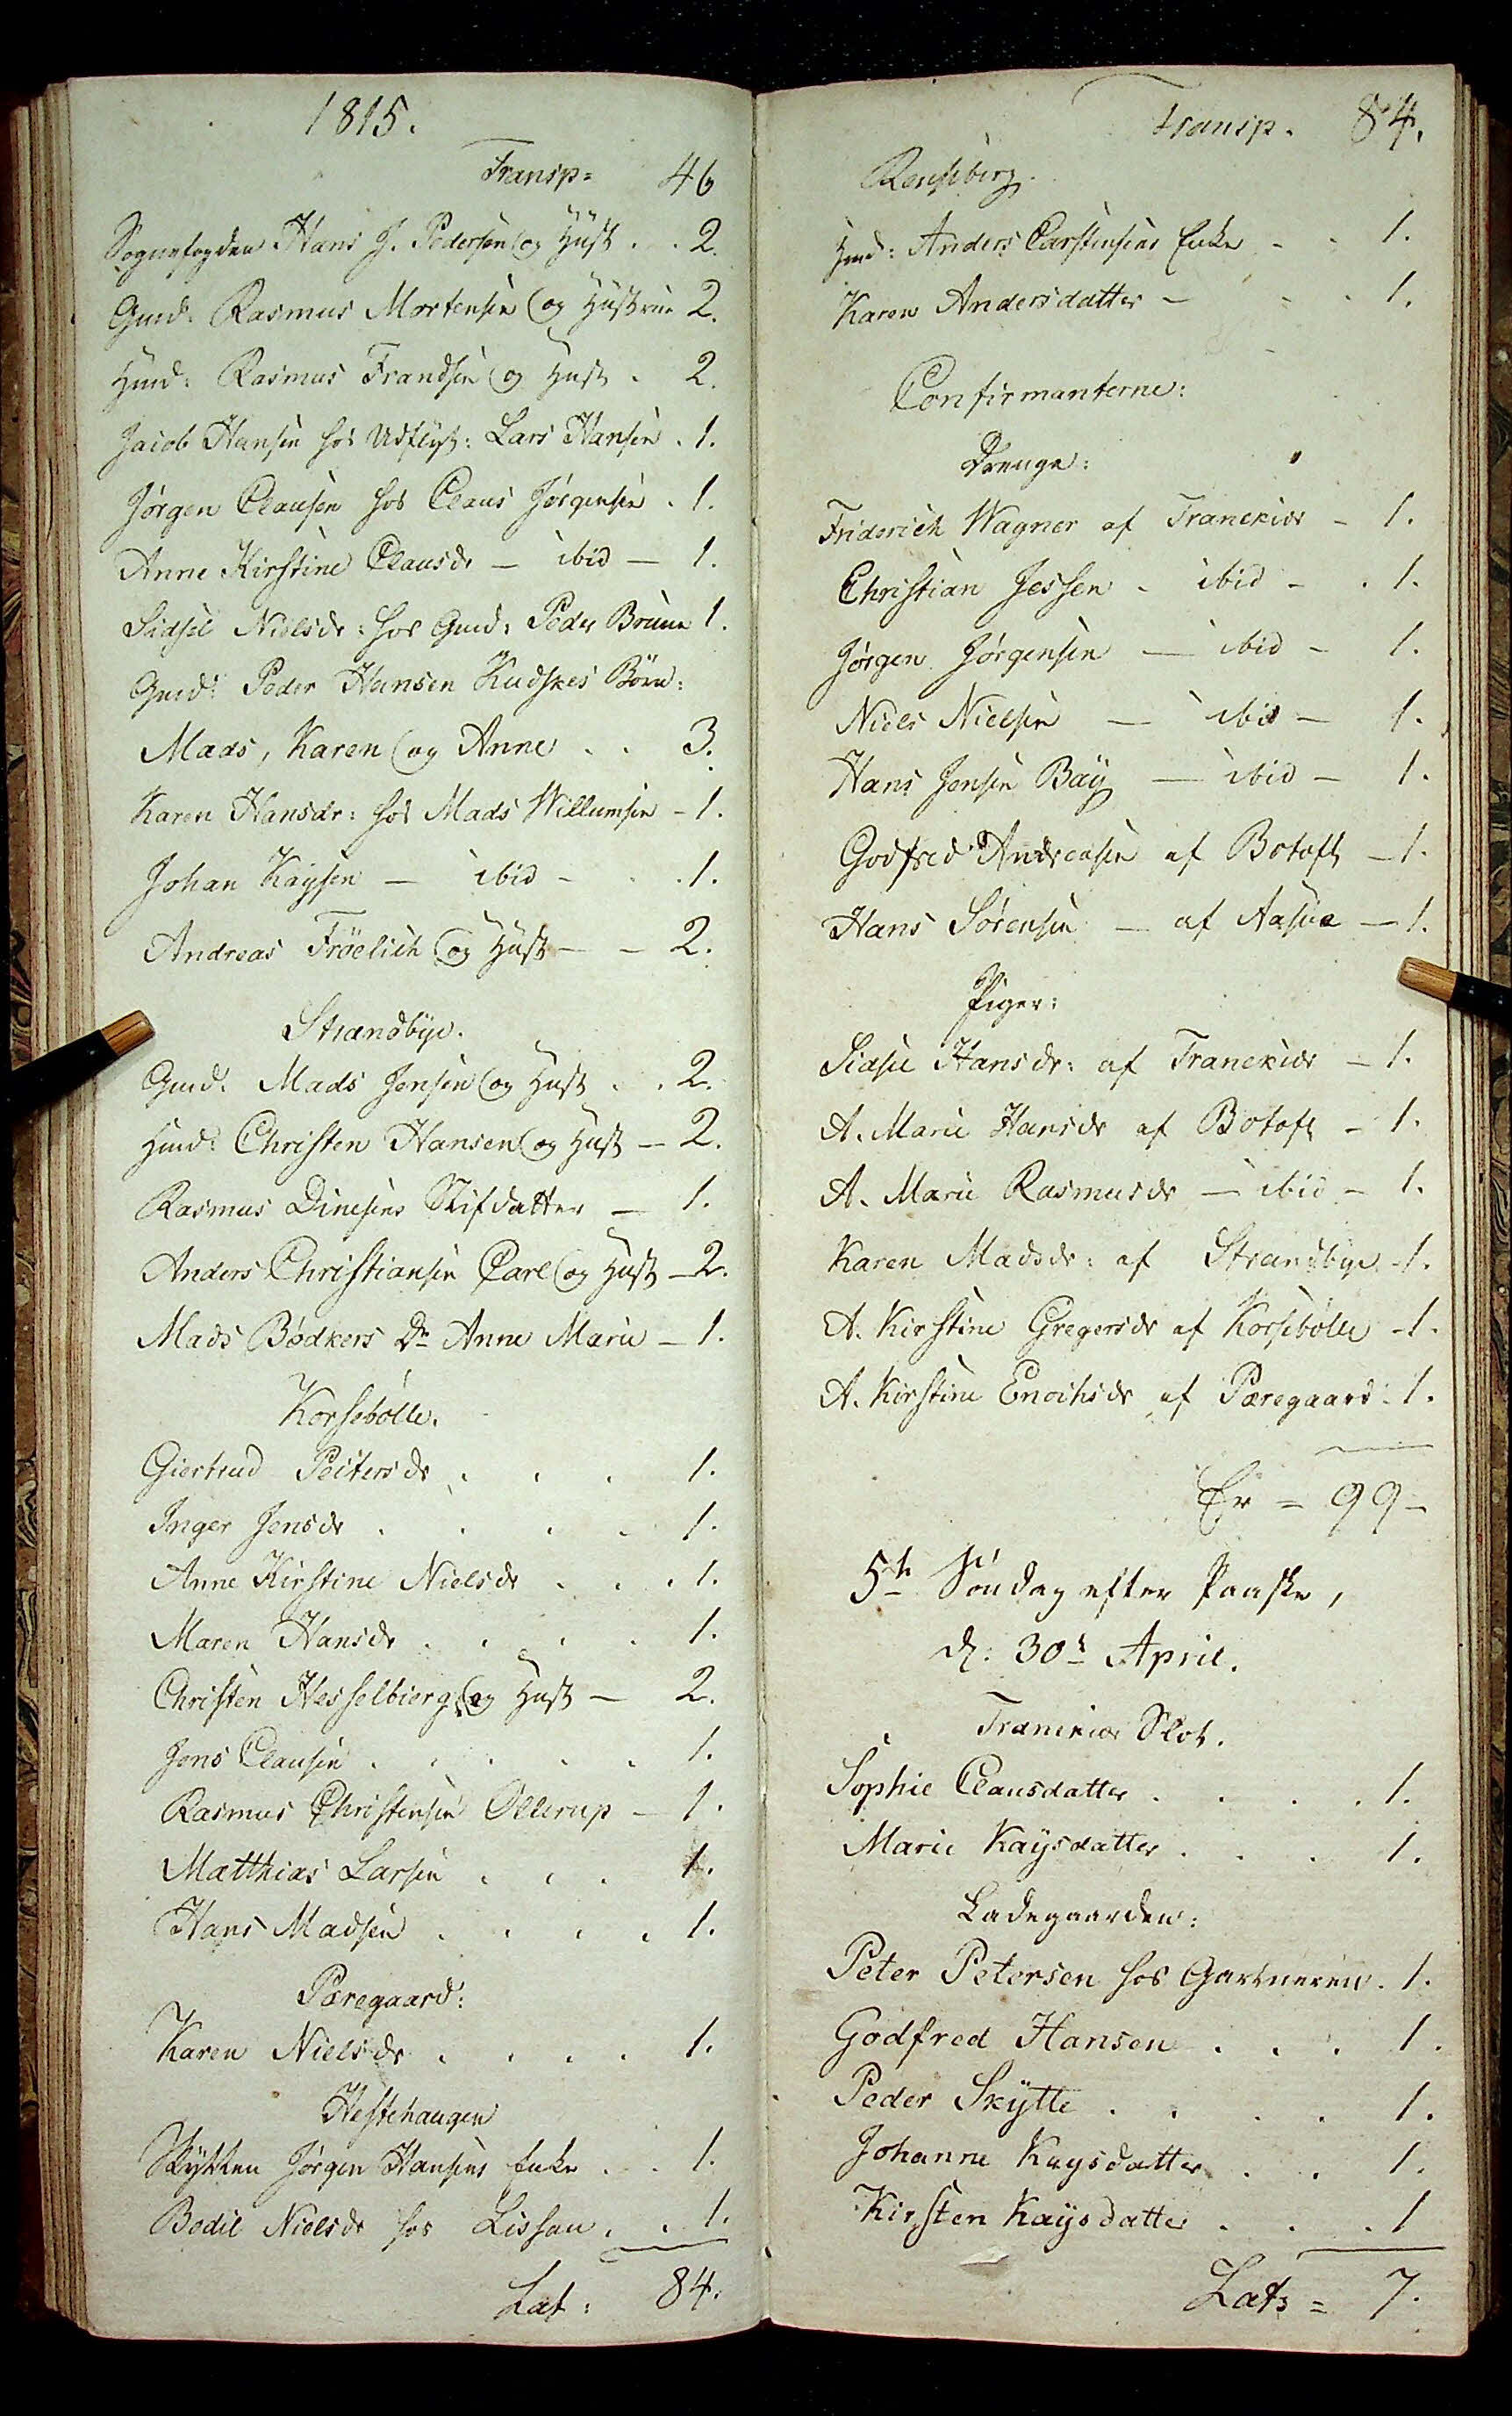1815.
Transp= 46
Sognefogden Hans J. Pedersen og Hust . . 2.
Gmd: Rasmus Mortensen og Hustrue 2.
Hmd: Rasmus Frandsen og Hust . 2.
Jacob Hansen hos Udflut: Lars Hansen . 1.
Jørgen Clausen hos Claus Jørgensen . 1.
Anne Kirstine Clausdr - ibid - 1.
Sidsel Nielsdr: hos Gmd: Peder Bruun 1.
Gmd: Peder Hansen Kudskes Børn:
Mads, Karen og Anne . . 3.
Karen Hansdr hos Mads Willumsen - 1.
Johan Kaysen - ibid - . .  1.
Andreas Frøclich og Hust - - 2.
Strandbye.
Gmd. Mads Jensen og Hust . . 2.
Hmd: Christen Hansen og Hust - - 2.
Rasmus Dinesens Stifdatter - 1.
Anders Christiansen Carl og Hust - 2.
Mads Bødkers Dr Anne Marie - 1.
Korsebølle.
Giertrud Peitersdr . . . 1.
Inger Jensdr . . . . 1.
Anne Kirstine Nielsdr . . . 1.
Maren Hansdr . . . . 1.
Christen Hesselbierg og Hust - 2.
Jens Clausen . . . . . 1.
Rasmus Christensen Ollerup - 1.
Matthias Larsen . . . 1.
Hans Madsen . . . 1.
Pæregaard:
Karen Nielsdr . . . . 1.
Hestehaugen
Skytten Jørgen Hansens Enke . . 1.
Bodil Nielsdr hos Lissau . . 1.
Lat: 84.
Transp . 84.
Renseberg
Hmd: Anders Carstensens Enke . . 1.
Karen Andersdatter - . . 1.
Confirmanterne:
Drenge:
Friderich Wagner af Tranekiær - 1.
Christian Jensen - ibid -  . 1.
Jørgen Jørgensen - ibid - 1.
Niels Nielsen - ibid - 1.
Hans Jensen Bay - ibid - 1.
Godfred Andreasen af Botoft - 1.
Hans Sørensen - af Aasøe - 1.
Piger:
Sidse Hansdr: af Tranekiær - 1.
A.  Marie Hansdr af Botoft - 1.
A. Marie Rasmusdr - ibid - 1.
Karen Madsdr: af Strandbyr - 1.
A. Kirstine Gregersdr af Korsebølle - 1.
A. Kirstine Enochsdr af Pæregaard - 1.
Er = 99-
5te Søndag efter Paaske,
d: 30te April.
Tranekiær Slot.
Sophie Clausdatter . . . . 1.
Marie Kaysdatter . . . 1.
Ladegaarden.
Peter Petersen hos Gartneren . 1.
Godfred Hansen . . . 1.
Peder Skytte . . . . 1.
Johanne Kaysdatter . . 1.
Kirsten Kaysdatter . . . 1
Lats= 7.
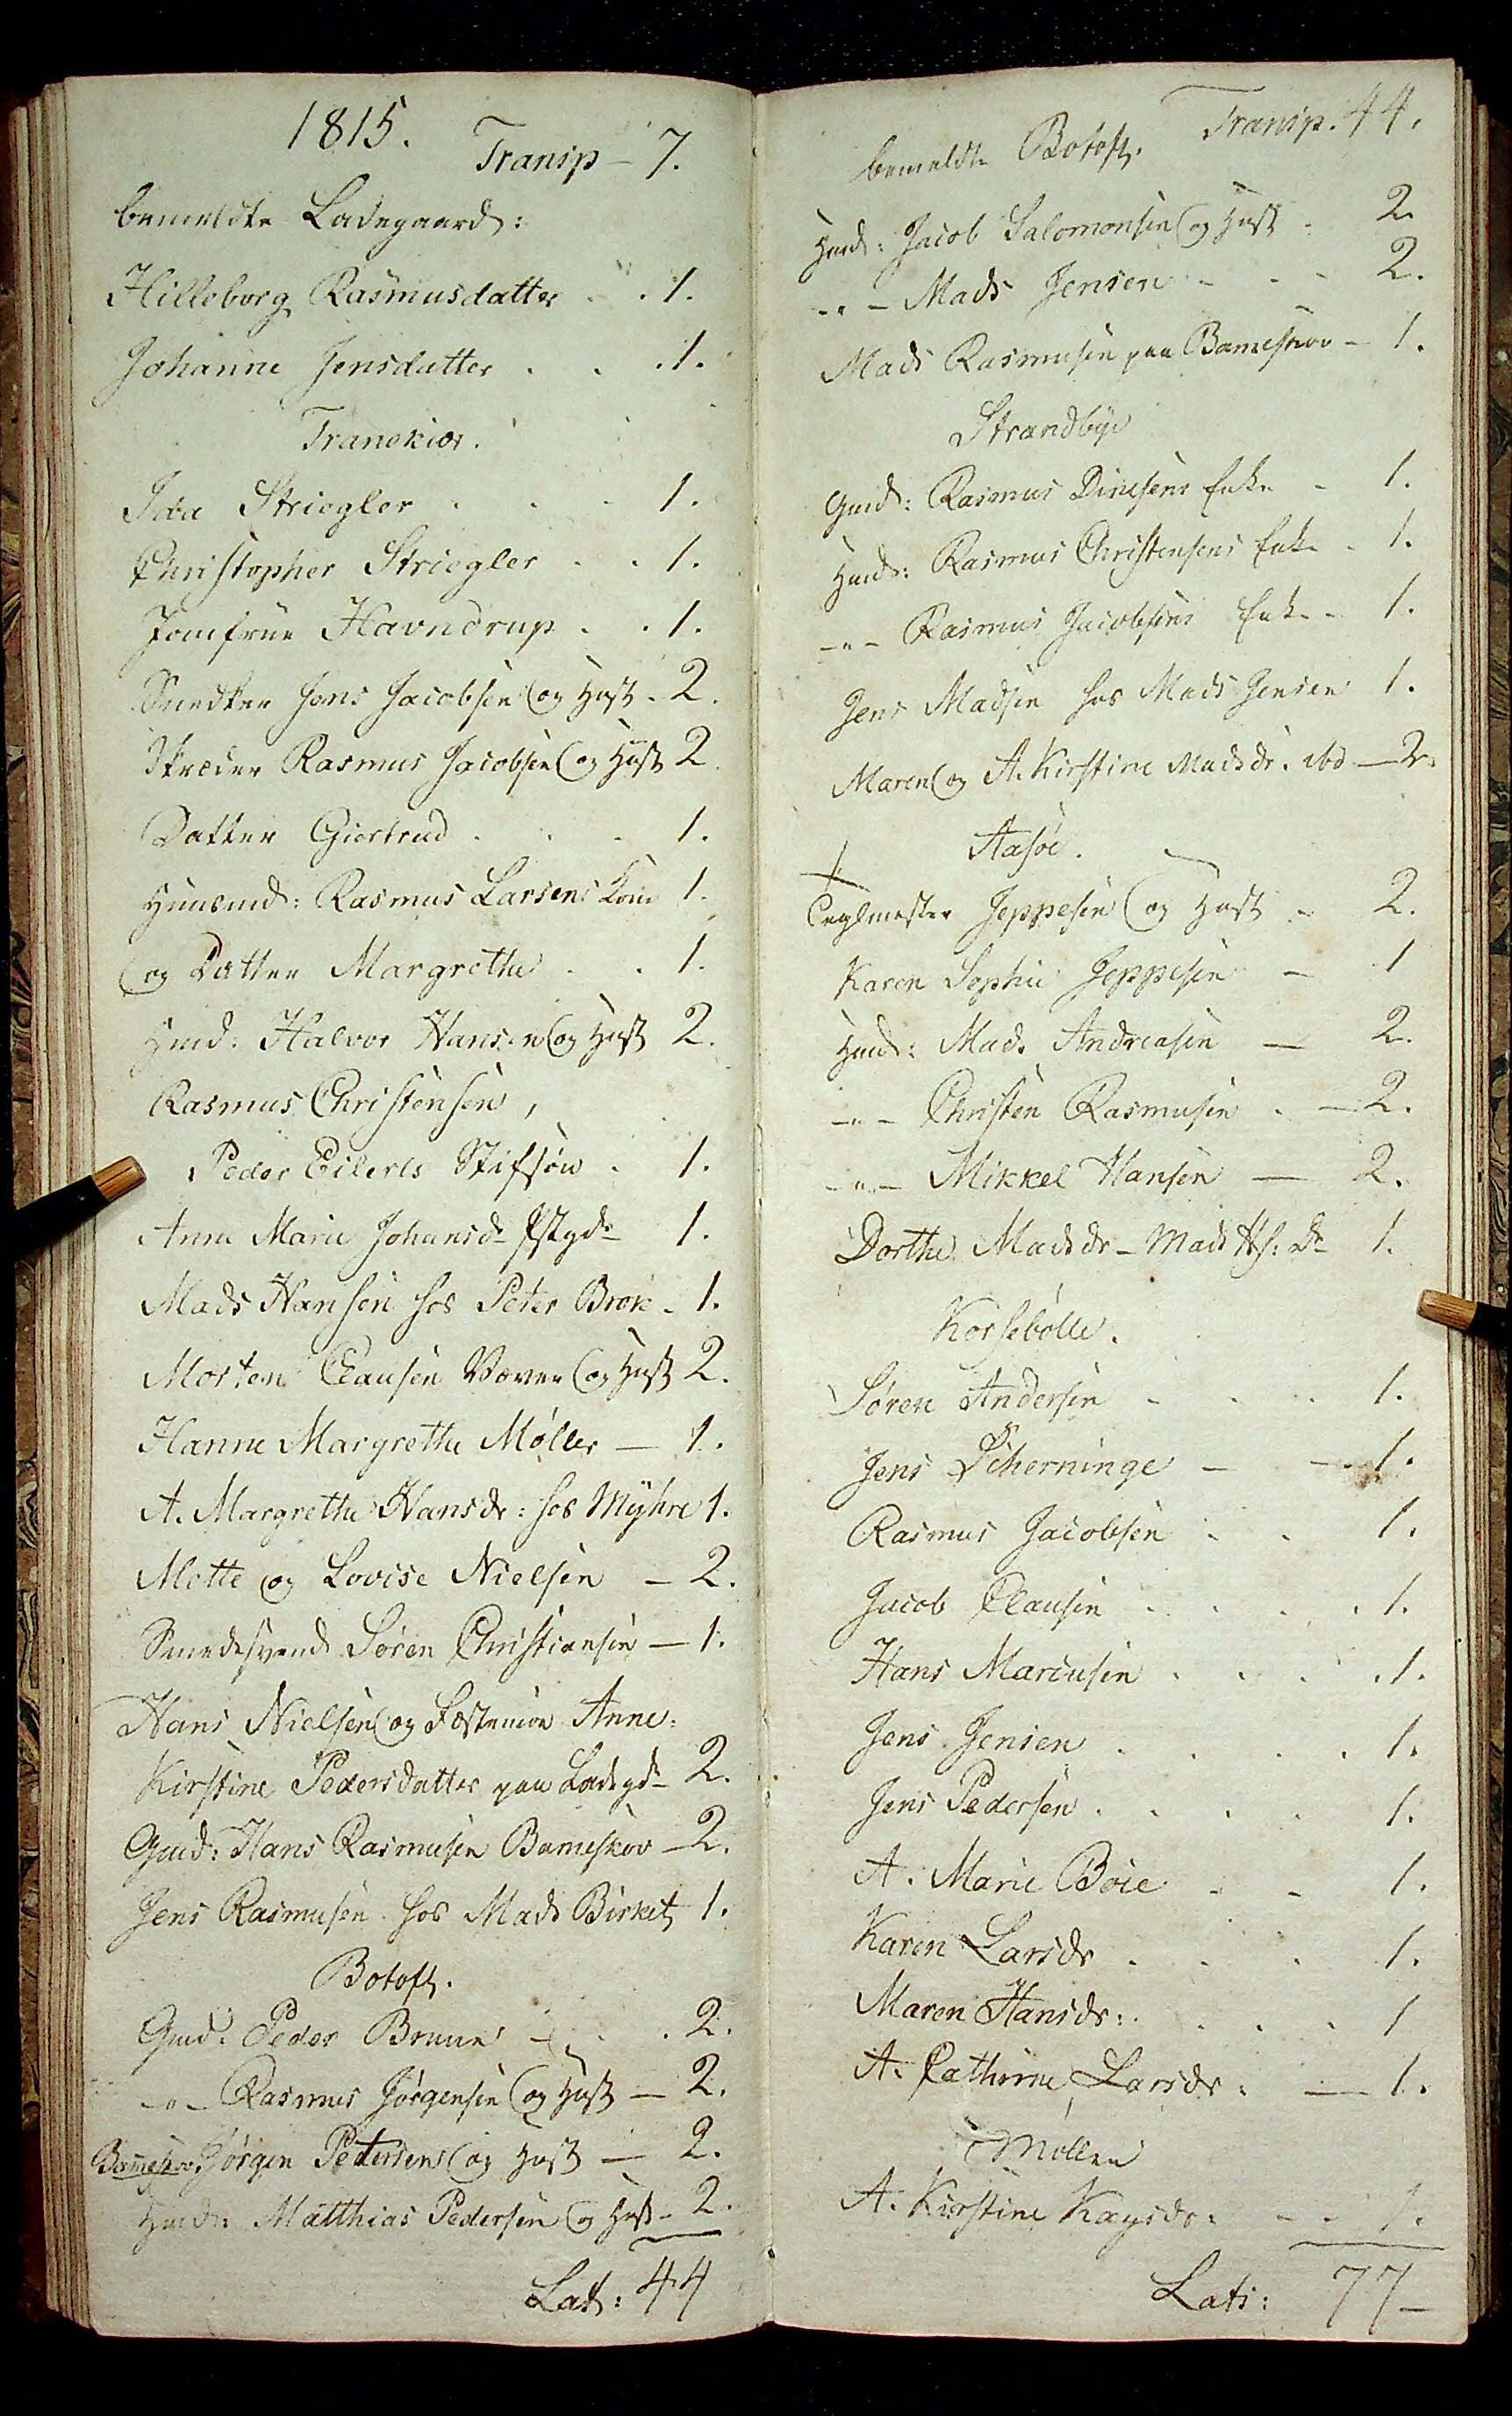1815.
Transp - 7.
Bemeldte Ladegaard:
Hilleborg Rasmusdatter . . 1.
Johanne Jensdatter . . . 1.
FTranekiær.
Ida Striegler . . . 1.
Christopher Striegler . . 1.
Jomfrue Havndrup . . 1.
Snedker Hans Jacobsen og Hust.  2.
Skræder, Rasmus Jacobsen og Hustr 2.
Datter Giertrud . . . 1.
Huusmd: Rasmus Larsens Kone 1.
og Datter Margrethe . . 1.
Hmd: Halvor Hansen og Hust 2.
Rasmus Christensen,
Peder Eilers Stifsøn . 1.
Anna Marie Johansdr Pstgdr 1.
Mads Hansen hos Peter Brok . 1.
Morten Clausen Væver og Hust  2.
Hanne Margrete Møller - 1
A. Margrethe Hansdr: hos Myhre 1.
Mette og Lovise Nielsen - 2.
Smedesvand Søren Christiansen - 1.
Hans Nielsen og Fæstemøe Anne:
Kirstine Pedersdatter paa Ladegdr - 2.
Gmd: Hans Rasmussen Bameskov - 2.
Jens Rasmussen hos Mads Birket 1.
Botoft.
Gmd: Peder Bruun - . . 2.
-"-  Rasmus Jørgensen og Hust - 2.
Bammeskov. Jørgen Petersens og hust - 2.
Hmd: Matthias Pedersen og Hust - 2.
Lat: 44
Transp . 44.
bermeldte Botoft.
Hmd: Jacob Salomonsen og Hust . 2.
-"- Mads Jensen . . . 2.
Mads Rasmussen paa Bameskov - 1.
Strandbye
Gmd: Rasmus Dinesens Enke - 1.
Hmd: Rasmus Christensens Enke . 1.
-"-  Rasmus Jacobsens Enke.
Jens Madsen hos Mads Jensens 1.
Maren og A: Kirstine Madsdr . ibid - 2
Aasøe.
Teglmester Jeppesen og Hust - 2.
Karen Sophie Jeppesen - 1
Hmd: Mads Andreasen - 2
-"- Christen Rasmussen . - 2.
-"- Mikkel Hansen - 2.
Dorthe Madsdr - Mads Hs: Dr 1.
Korsebølle.
Søren Andersen - . . 1.
Jens Scherninge - - . 1.
Rasmus Jacobsen . . 1.
Jacob Clausen . . . . 1.
Hans Marcusen . . . . 1.
Jens Jensen . . . . 1.
Jens Pedersen . . . . 1.
A. Marie Boie - - 1.
Karen Larsdr . . . 1.
Maren Hansdr: . . . . 1
A. Cathrine Larsdr . - 1.
Møllen
A. Kirstine Kaysdr: - - 1.
Lats: 77-